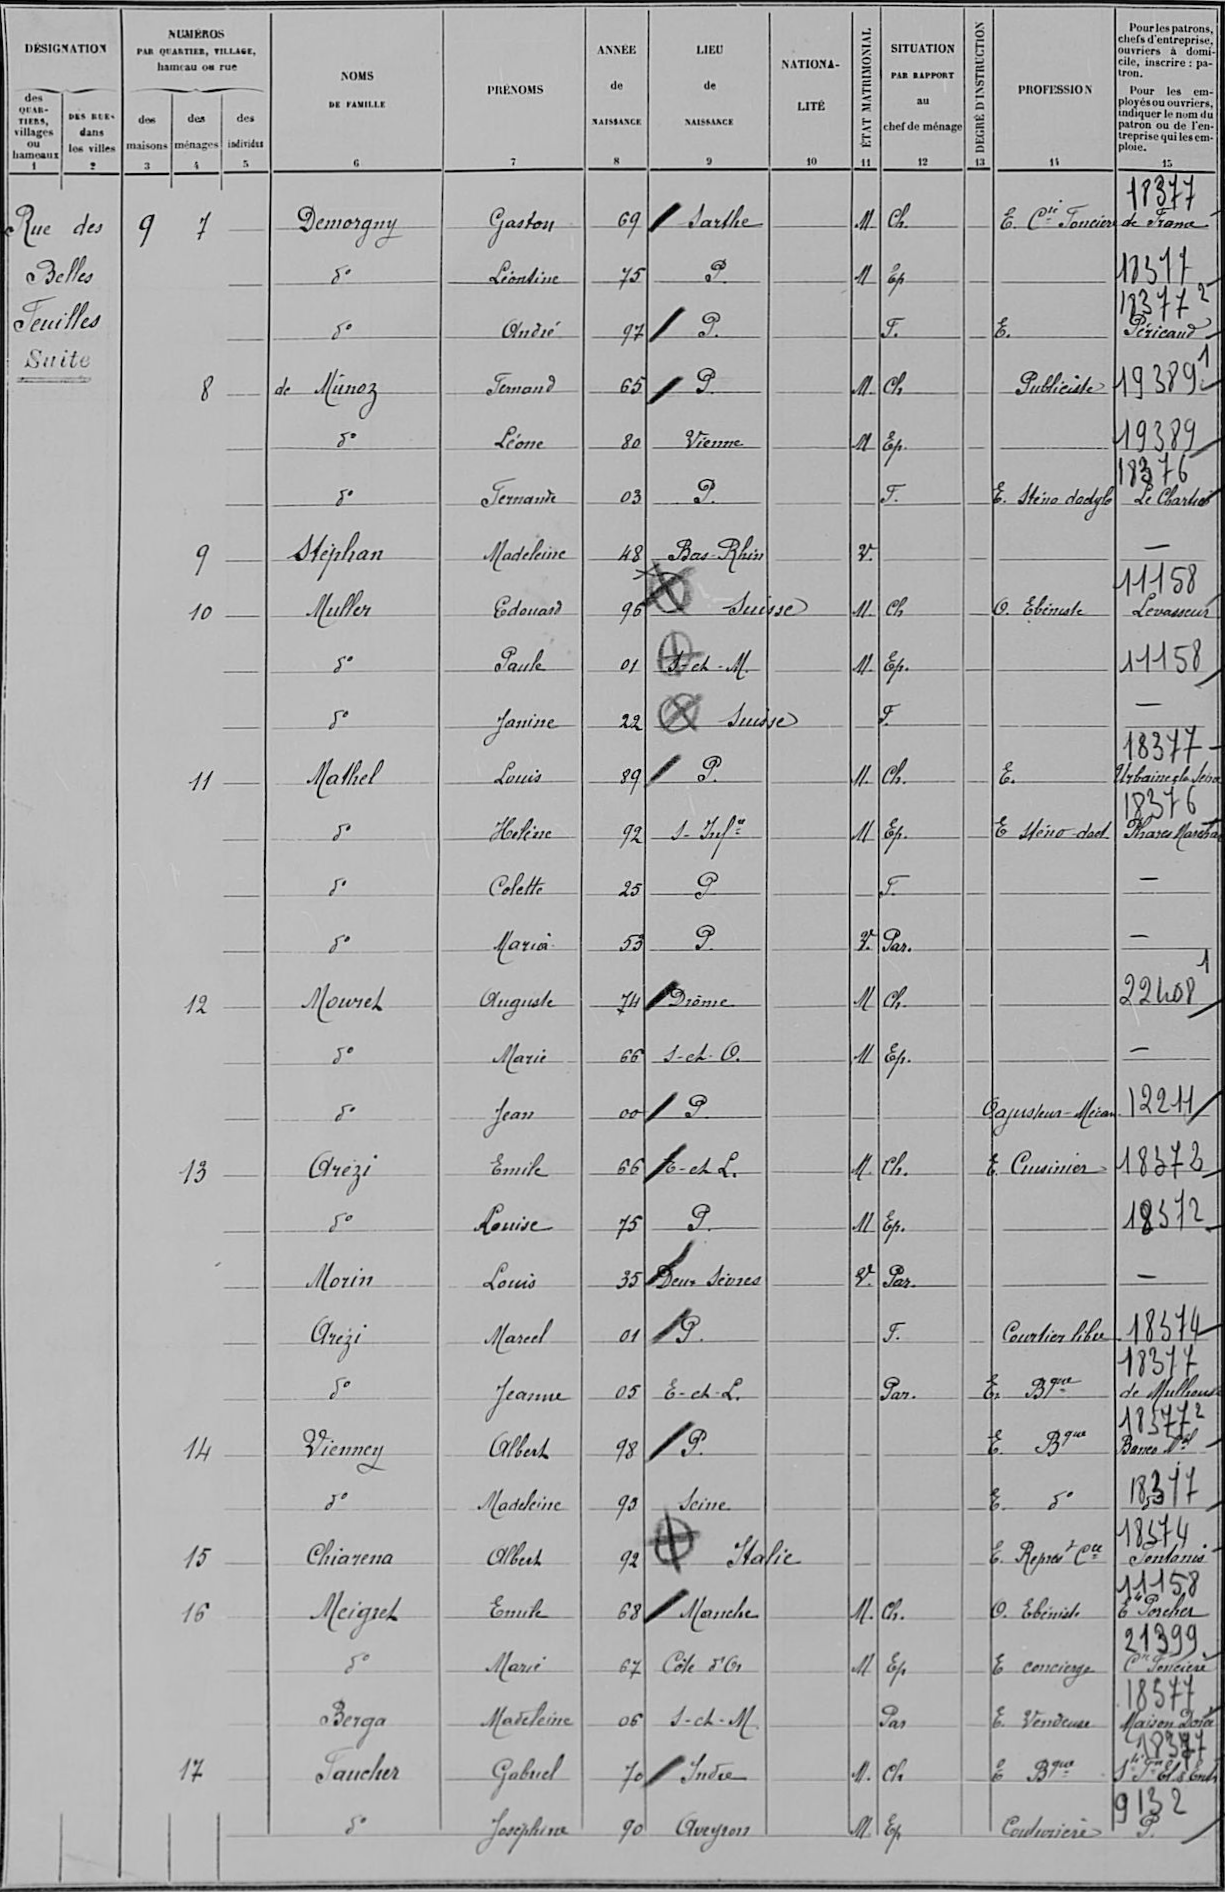Demorgny/Gaston/69/Sarthe/¤/M/Ch /¤/E C Fonciere/de France?18377
d°/Léontine/75/P /¤/M/Ep/¤/¤/18377
d°/André/97/P /¤/¤/F /¤/E /Péricaud 18377
de Munoz/Fernand/65/P /¤/M/Ch/¤/Publiciste/193891
d°/Léone/80/Vienne/¤/M/Ep/¤/¤/19389
d°/Fernande/03/P /¤/¤/F /¤/E sténo dactylo/Le Chartier ?18376
Stéphan/Madeleine/48/Bas-Rhin/¤/V /¤/¤/¤/11158
Muller/Edouard/96/Suisse/¤/M /Ch/¤/O Ebeniste /Levasseur ?11158
d°/Paule/01/S ch M/¤/M/Ep /¤/¤/11158
d°/Janine/22/Suisse/¤/¤/F /¤/¤/18377
Mathel/Louis/89/P /¤/M /Ch /¤/E /Urbaine la Seine ?18377
d°/Hélène/92/S-Inf/¤/M/Ep /¤/E sténo dact/Phares Marchan ?18376
d°/Colette/25/P/¤/¤/F /¤/¤/¤
d°/Maria/53/P /¤/V /Par /¤/¤/¤
Mourel/Auguste/74/Drôme/¤/M/Ch/¤/¤/22408
d°/Marie/66/s-ch-O/¤/M/Ep /¤/¤/¤
d°/Jean/00/P /¤/¤/¤/¤/O ajusteur Mécan/12211
Arezi/Emile/66/E-ch L /¤/M /Ch /¤/E Cuisinier/18372
d°/Louise/75/P /¤/M/Ep /¤/¤/18372
Morin/Louis/35/Deux Sèvres/¤/V /Par /¤/¤/¤
Arézi/Mareel/01/P /¤/¤/F /¤/Courtier libre/18374
d°/Jeanne/05/E-ch L /¤/¤/Par /¤/E Bque de Mulhouse/?18377
Vienney/Albert/98/P /¤/¤/¤/¤/E Bque/Banco Nal ?18377
d°/Madeleine/95/Seine/¤/¤/¤/¤/E d°/18377
Chiarena/Albert/92/Italie/¤/¤/¤/¤/E Represt Cce/Fontanis ?18374
Meigret/Emile/68/Manche/¤/M /Ch /¤/O Ebeniste /Ets Porcher ?11158
d°/Marie/67/Cote d'Or/¤/M/Ep/¤/E concierge /C Fonciere ?21399
Berga/Madeleine/06/S-ch-M/¤/¤/Par/¤/E Vendeuse/Maison Dorée ?18377
Faucher/Gabriel/70/Indre/¤/M /Ch/¤/E Bque/Ste Gale Ets Ents ?18377
d°/Joséphine/90/Aveyron/¤/M /Ep/¤/Couturière/P ?9132
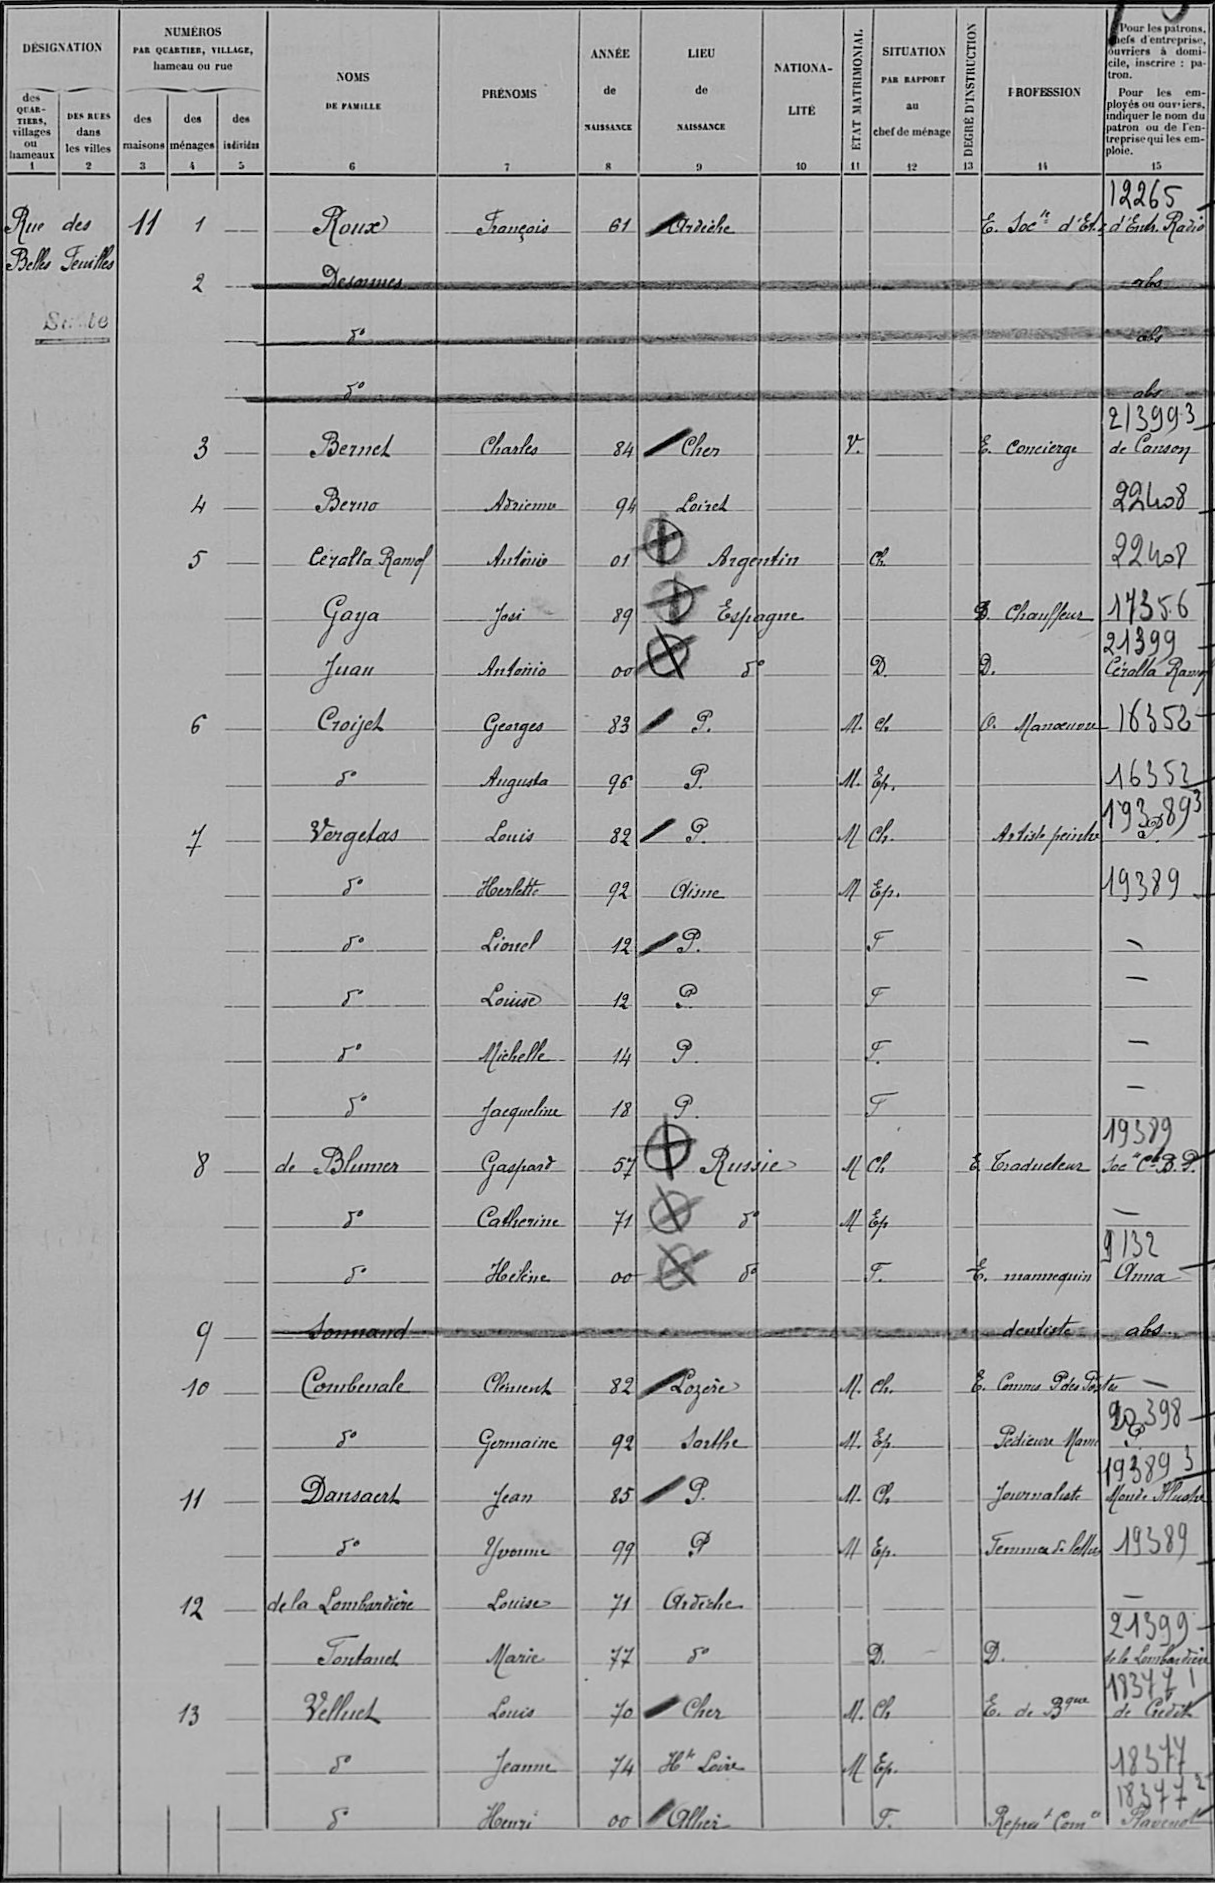Roux/François/61/Ardèche/¤/¤/¤/¤/E Soc d'Et d'Entr Radio/?12265
Desannes/¤/¤/¤/¤/¤/¤/¤/¤/abs
d°/¤/¤/¤/¤/¤/¤/¤/¤/abs
d°/¤/¤/¤/¤/¤/¤/¤/¤/abs
Bernet/Charles/84/Cher/¤/V /¤/¤/E Concierge/de Canson?213993
Berno/Adrienne/94/Loiret/¤/¤/¤/¤/¤/22408
Ceralta Raniof/Antonio/01/Argentin/¤/¤/Ch /¤/¤/22408
Gaya/José/89/Espagne/¤/¤/¤/¤/D Chauffeur/17356
Juan/Antonio/00/d°/¤/¤/D /¤/D /Céralta Raniof?21399
Croijet/Georges/83/P /¤/M /ch /¤/O Manoeuvre/16352
d°/Augusta/96/P /¤/M /Ep /¤/¤/16352
Vergelas/Louis/82/P /¤/M/ch /¤/artiste peintre/193893
d°/Herlette/92/Aisne/¤/M/Ep /¤/¤/19389
d°/Lionel/12/P /¤/¤/F/¤/¤/¤
d°/Louise/12/P /¤/¤/F/¤/¤/¤
d°/Michelle/14/P /¤/¤/F/¤/¤/¤
d°/Jacqueline/18/P /¤/¤/F/¤/¤/¤
de Blumer/Gaspard/57/Russie/¤/M/Ch/¤/E Traducteur/Soc C B P ?19389
d°/Catherine/71/d°/¤/M/Ep/¤/¤/¤
d°/Hélène/00/d°/¤/¤/F/¤/E mannequin/Anna ?9132
Sonnand/¤/¤/¤/¤/¤/¤/¤/dentiste/abs
Combenale/Clément/82/Lozère/¤/M /Ch /¤/E Commis P des Postes/20398
d°/Germaine/92/Sarthe/¤/M/Ep/¤/Pedicure Manuc/P ?20398
Dansaert/Jean/85/P /¤/M/Ch/¤/Journaliste/Monde illustre?193893
d°/Yvonne/99/P/¤/M/Ep /¤/Femme de lettres/19389
de la Lombardière/Louise/71/Ardèche/¤/¤/¤/¤/¤/21399
Fontanet/Marie/77/d°/¤/¤/D /¤/D /de la lombardière ?21399
Velluck/Louis/70/Cher/¤/M /Ch/¤/E de Bque de crédit/?183771
d°/Jeanne/74/Hte Loire/¤/M/Ep /¤/¤/18377
d°/Henri/00/Allier/¤/¤/F /¤/Repres Com/Flavenot ?18377